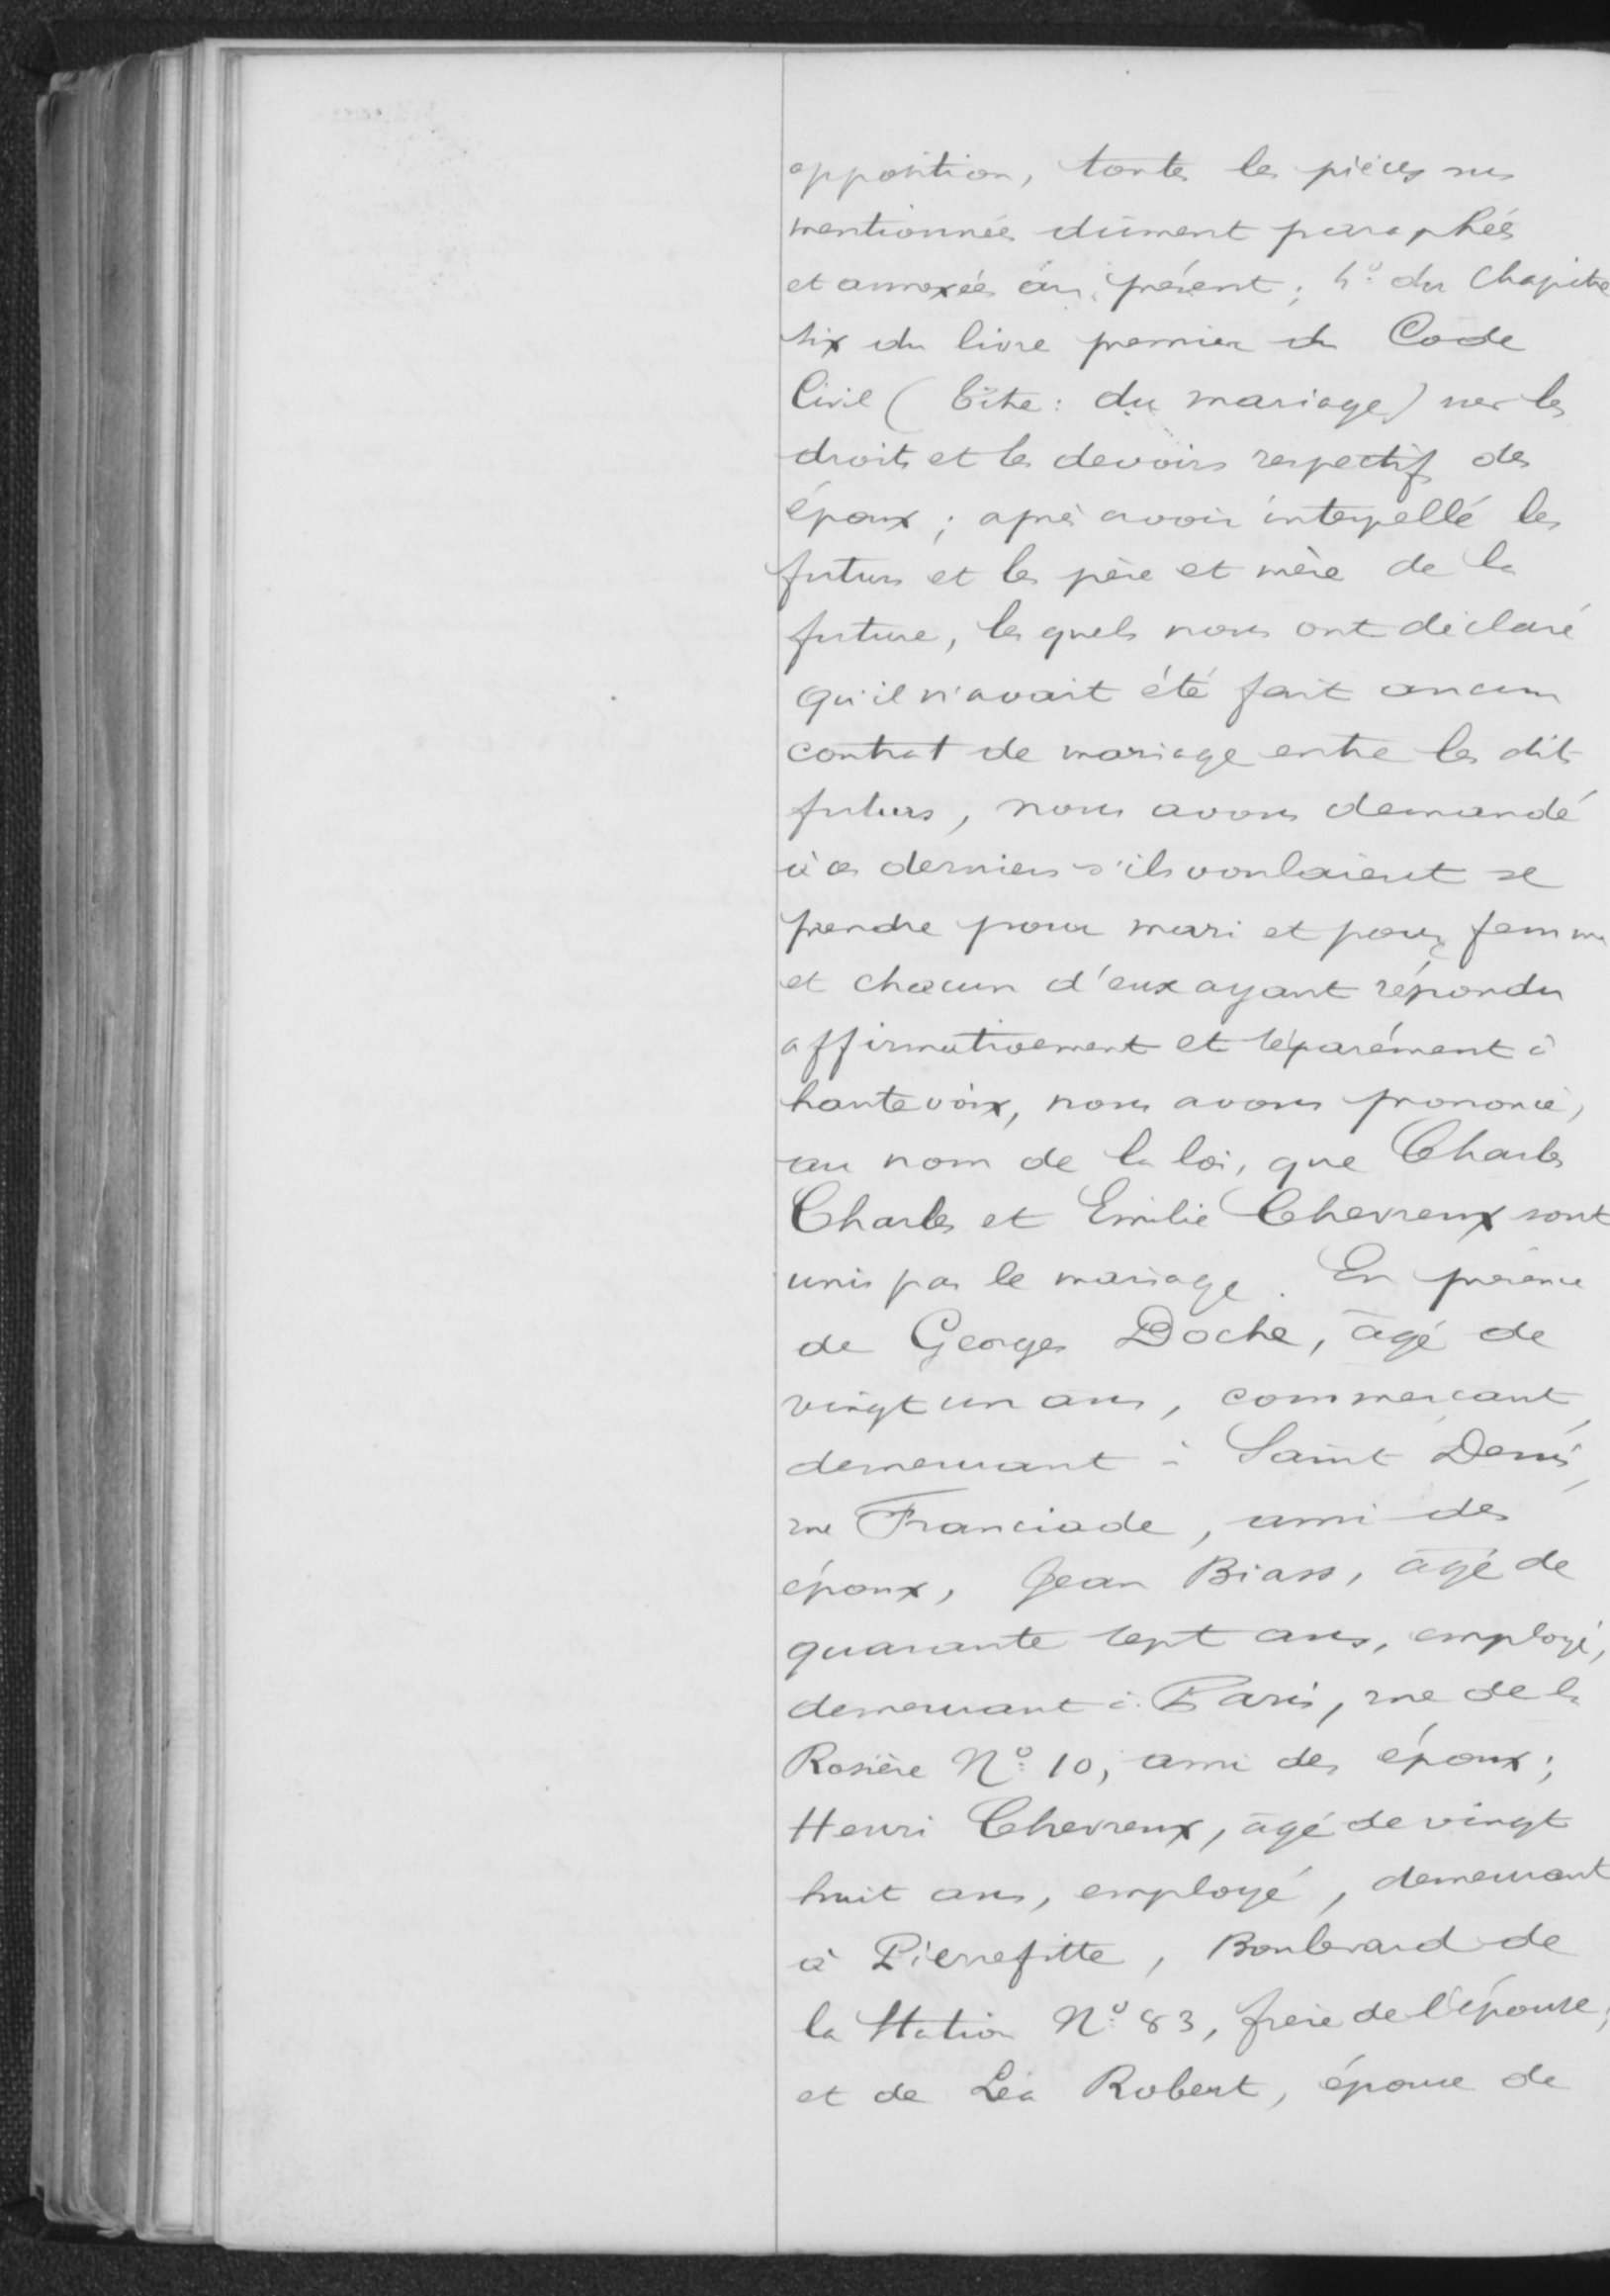opposition, toutes les pièces ne
mentionnée dûment paraphées
et annexées en présent; 4°du chapitre
six du livre premier et Code
Civil (Titre: du mariage) sur les
droits et le devoirs respectifs des
époux; après avoir interpellé les
futurs et les père et mère de la
future, lesquels nous ont déclaré
qu'il n'avait été fait aucun
contrat de mariage entre les dits
futurs, nous avons demandé
à ces derniers s'ils voulaient se
prendre pour mari et pour femme
et chacun d'eux ayant répondu
affirmativement et séparément à
haute voix, nous avons prononcé,
au nom de la loi, que Charles
Charles et Emilie Chevreux sont
unis par le mariage. En présence
de Georges Doche, âgé de
vingt un ans, commerçant,
demeurant à Saint Denis,
rue Franciade, ami des
époux, Jean Biass, âgé de
quarante sept ans, employé
demeurant à Paris, rue de la
Rosière N°10, ami des époux;
Henri Chevreux, âgé de vingt
huit ans, employé, demeurant
à Pierrefitte, Boulevard de
la Nation N°: 83, frère de l'épouse;
et de Léa Robert, époux de
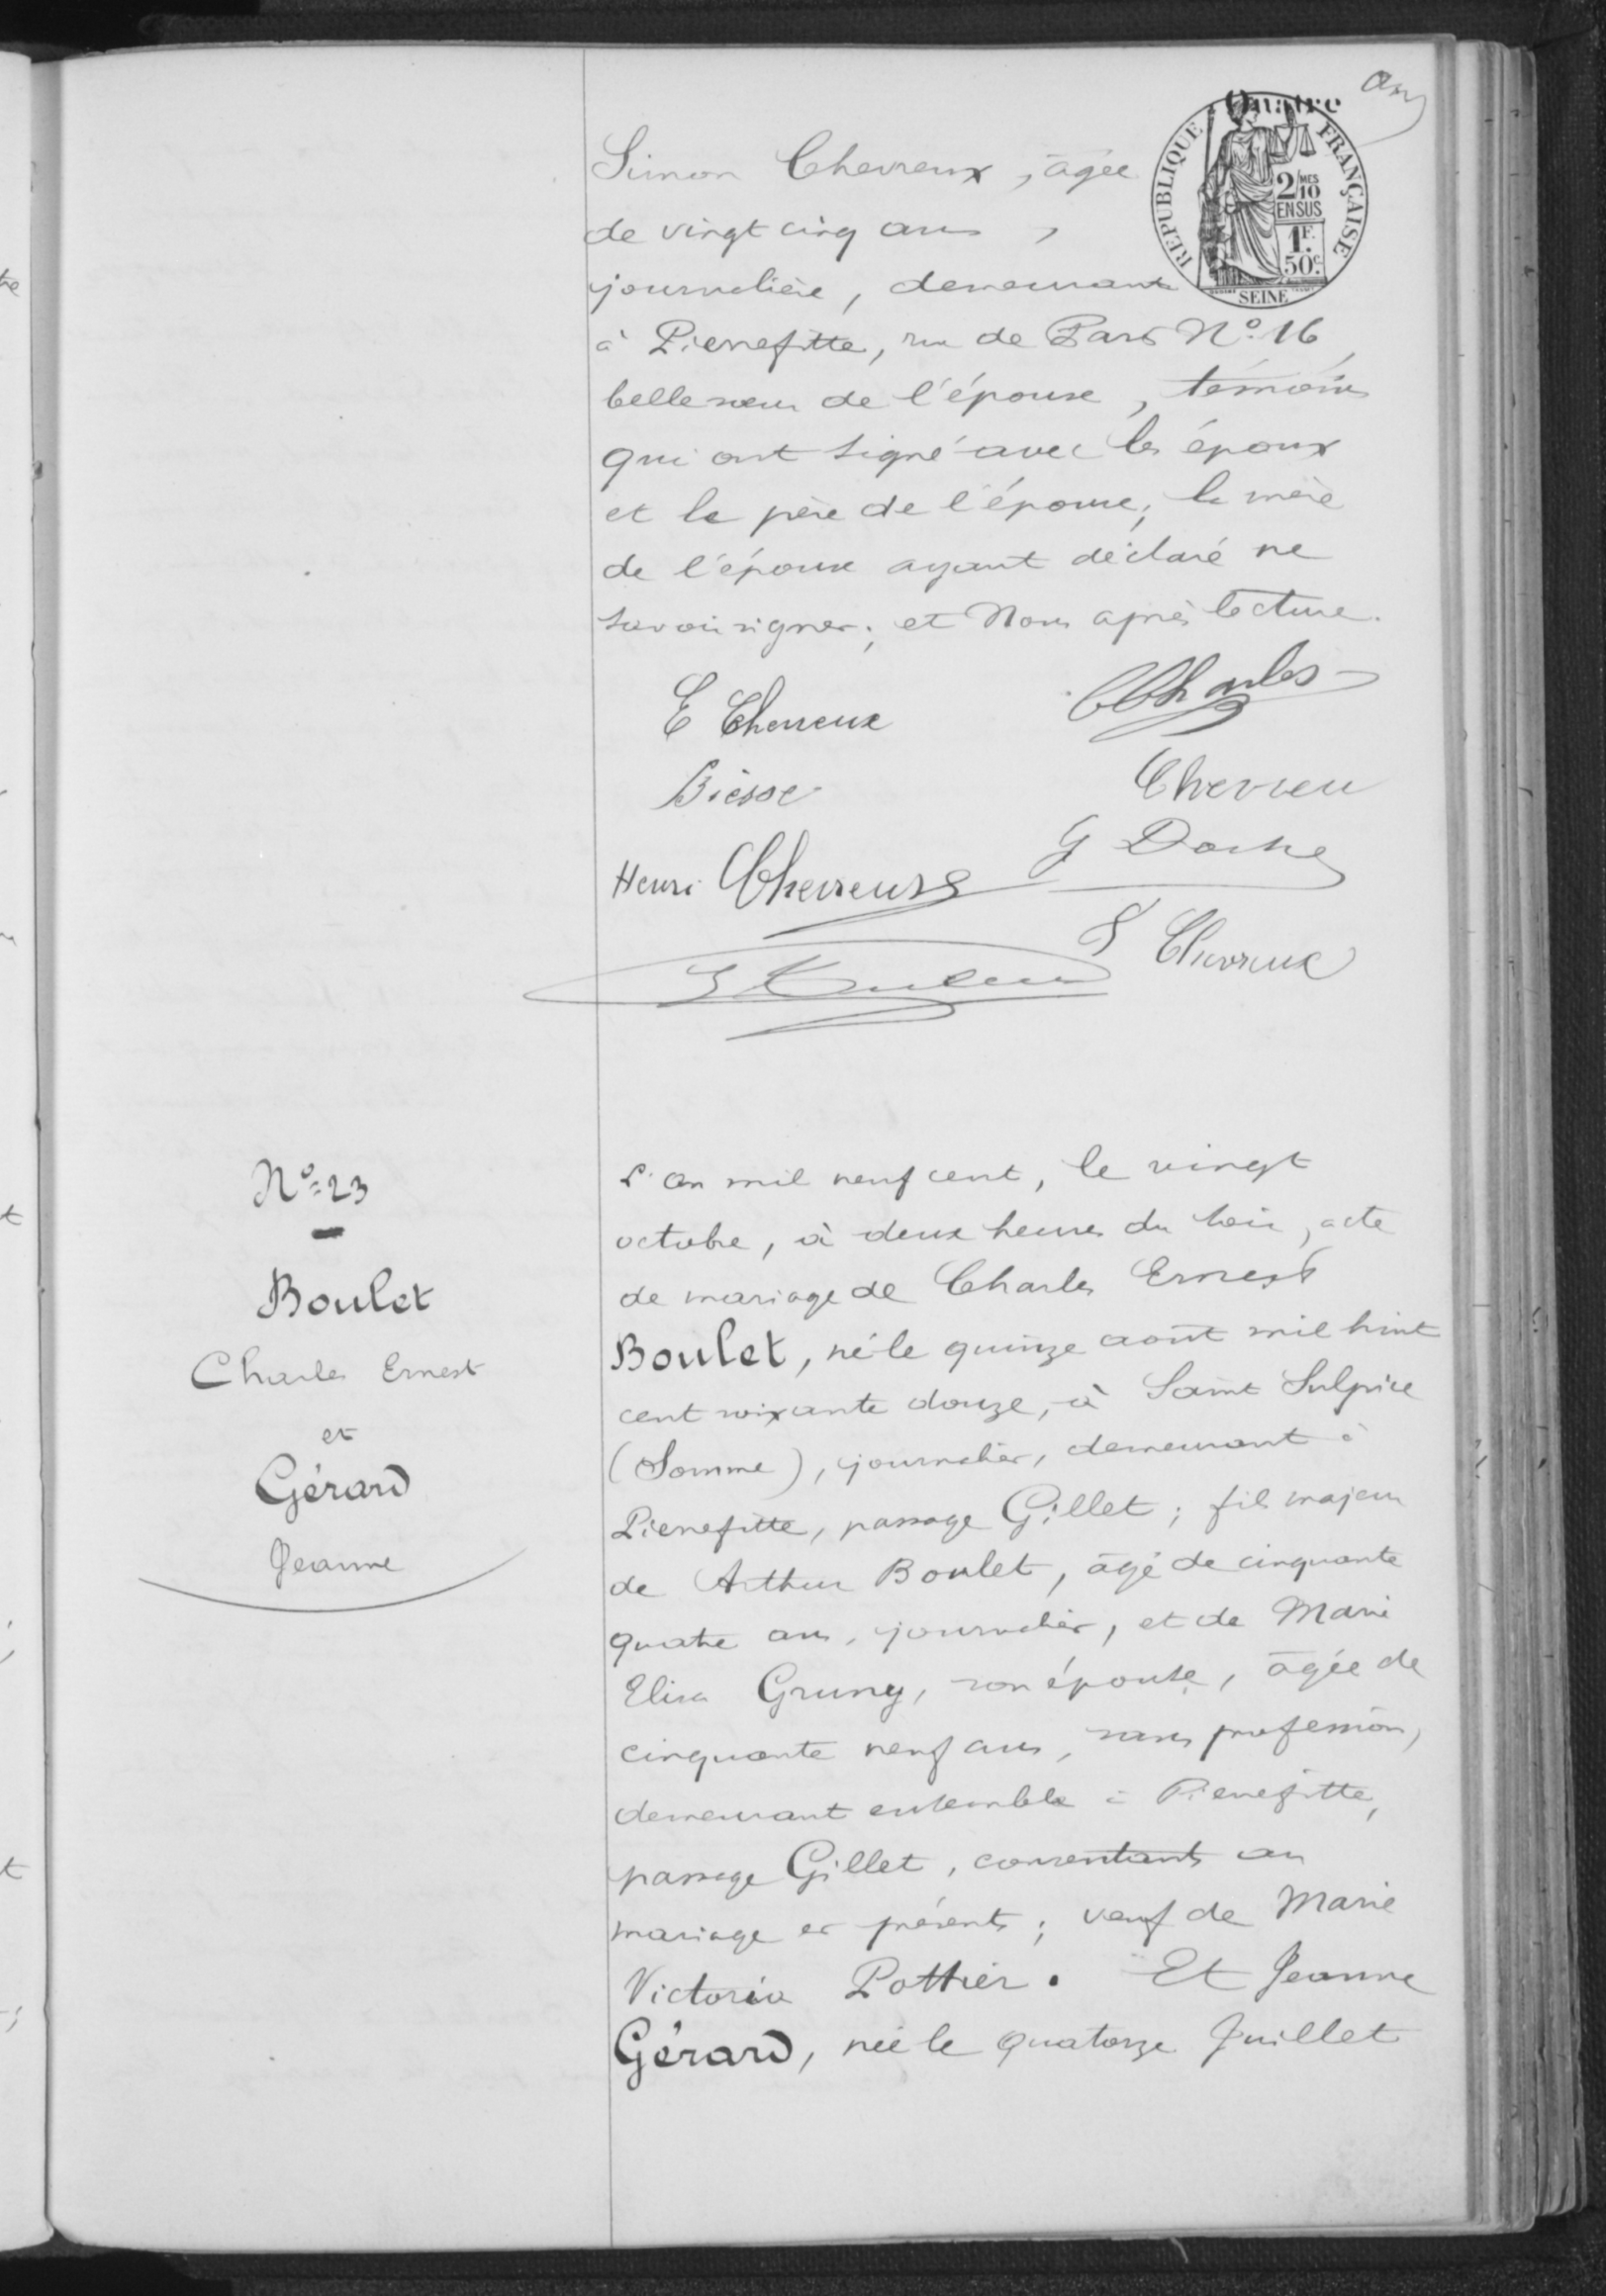Simon Chevreux, âgée
de vingt cinq ans,
journalière, demeurant
à Pierrefitte, rue de Part N°16,
belle soeur de l'épouse, témoins
qui ont signé avec les époux
et le père de l'épouse; la mère
de l'épouse ayant déclaré ne
savoir signer; et Nous après lecture
N°:23
Boulet
Charles Ernest
et
Gérard
Jeanne
L'an mil neuf cent, le vingt
octobre,
à deux heures du soir, acte
de mariage de Charles Ernest
Boulet né le quinze août mil huit
cent soixante douze, à Saint Sulpice
(Somme), journelier, demeurant à
Pierrefitte, passage Gillet; fils majeur
de Arthur Boulet, âgé de cinquante
quatre ans, journalier, et de Marie
Elise Gruny, son épouse, âgée de
cinquante neuf ans, sans profession,
demeurant ensemble à Pierrefitte,
passage Gillet, consentant au
mariage et présents; veuf de Marie
Victoria Pottier. Et Jeanne
Gérard, née le quatorze Guillet